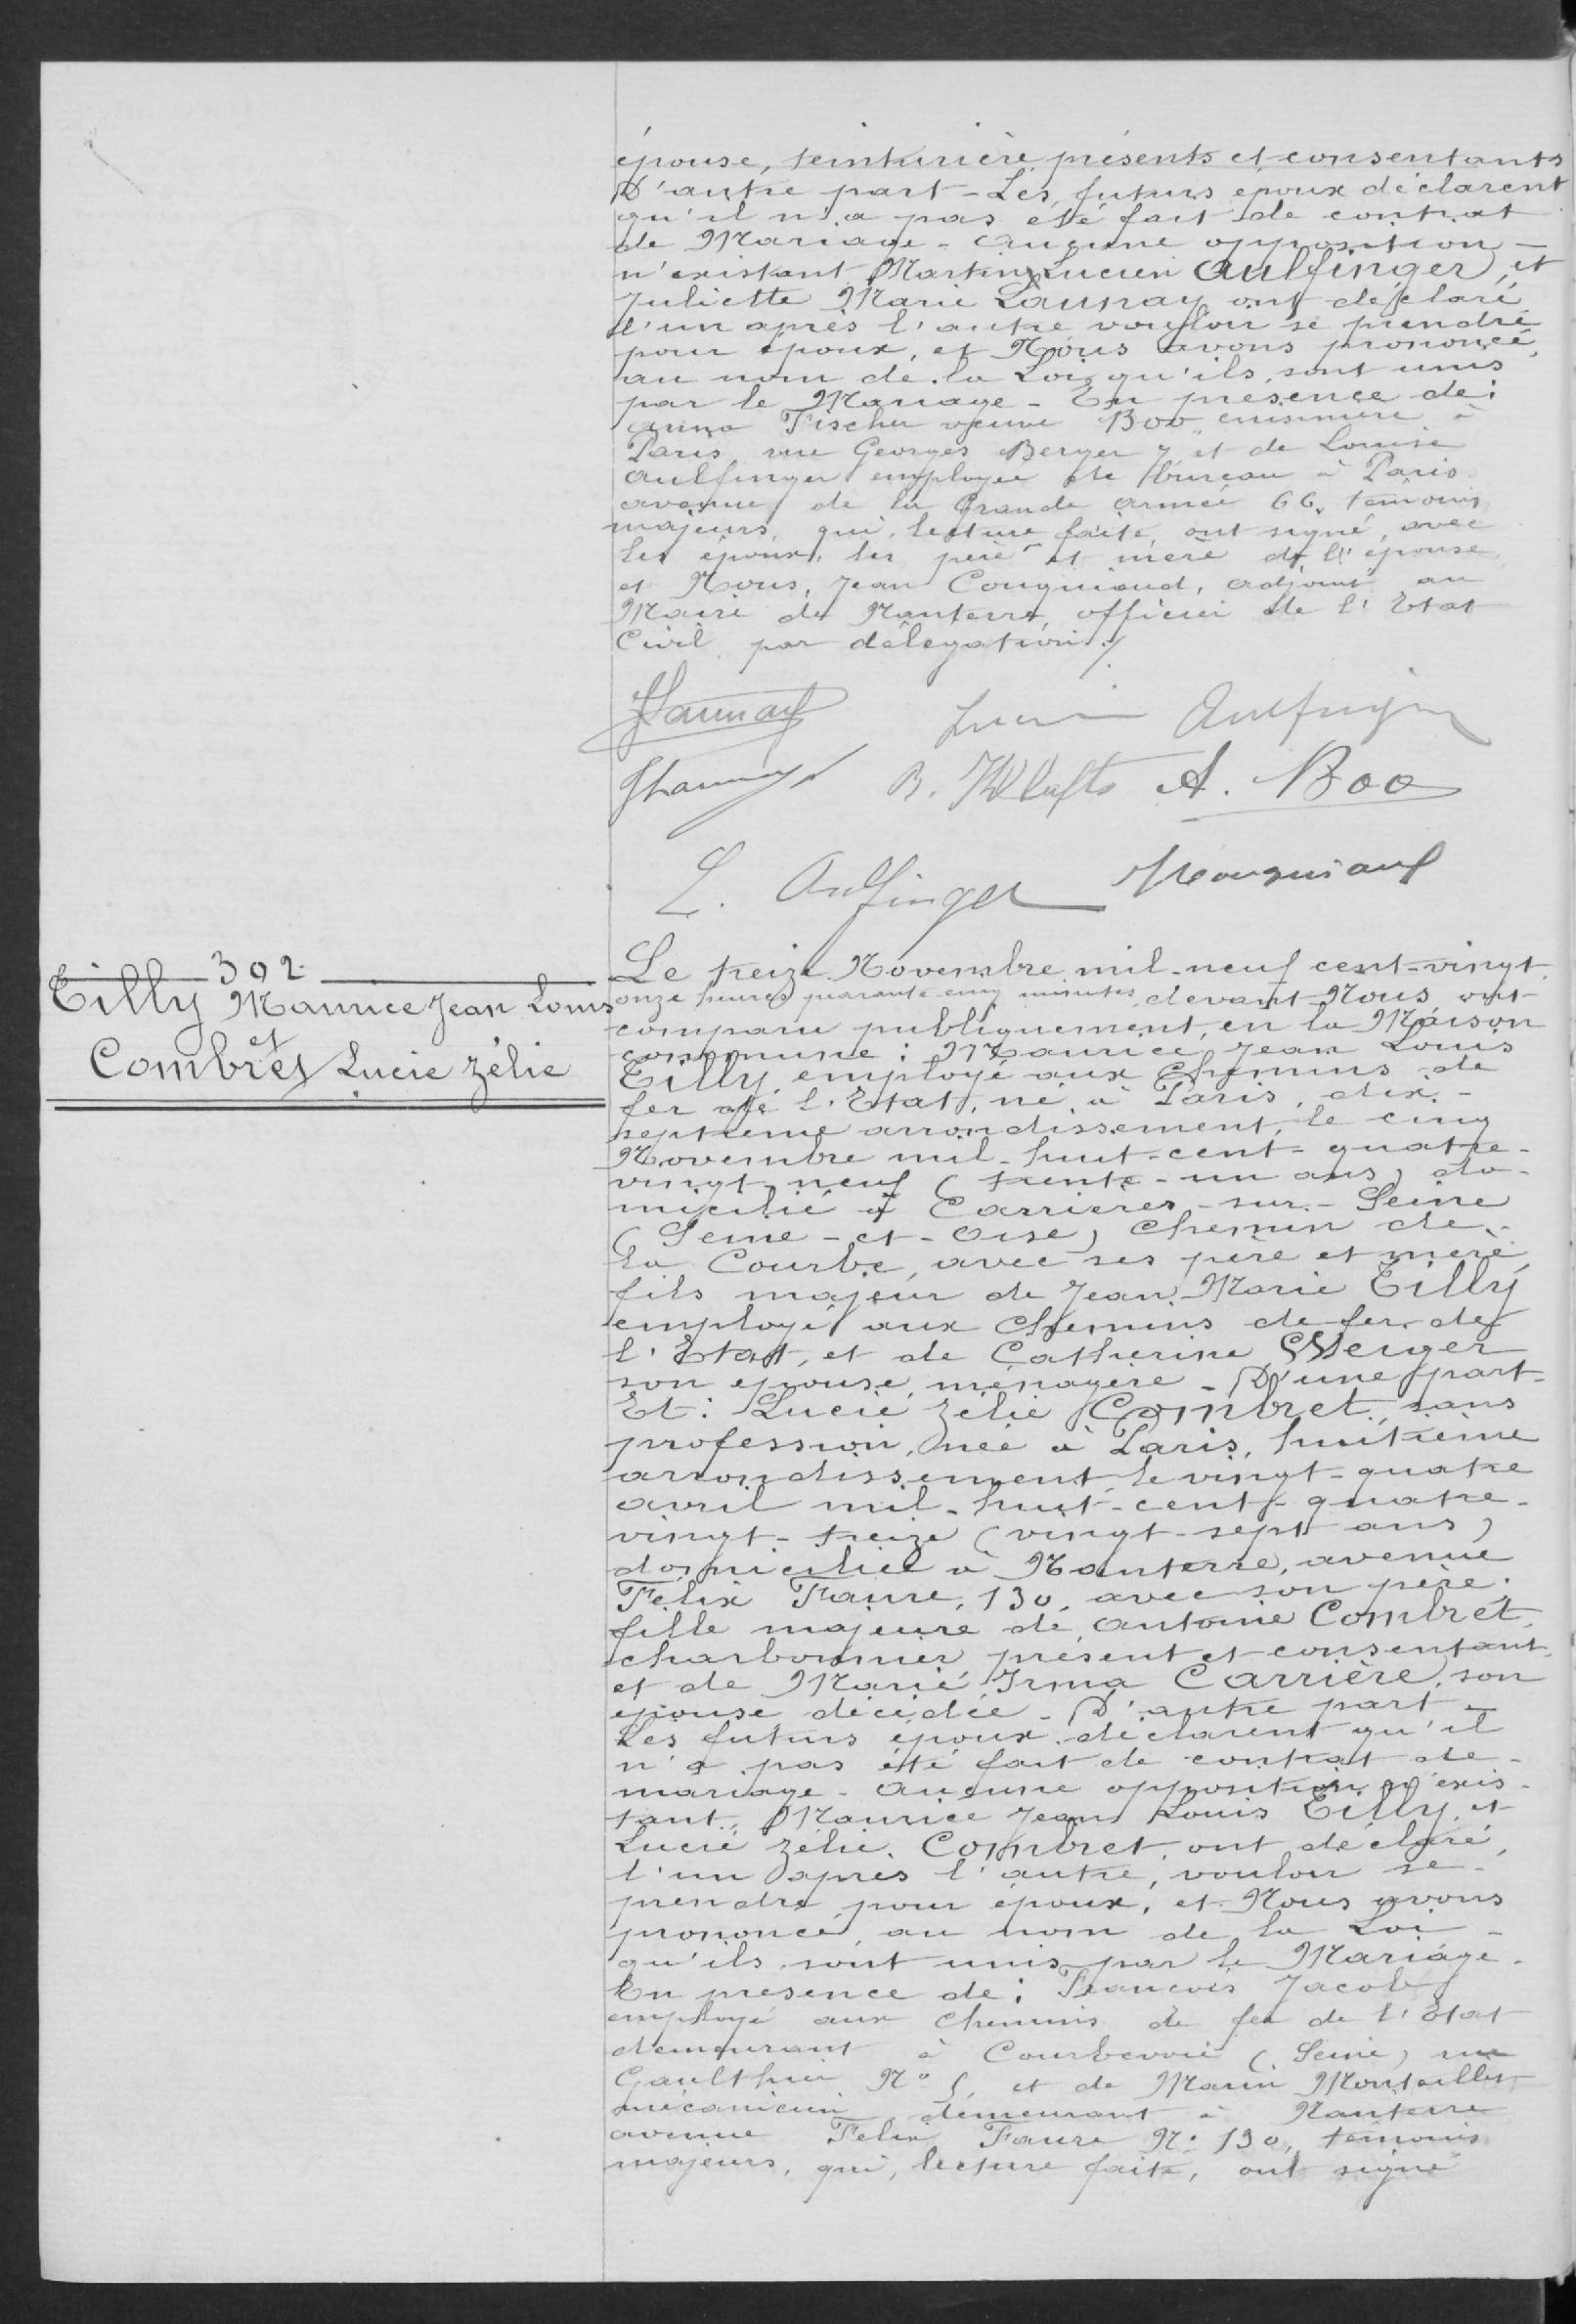épouse, teinturière présents et consentant
D'autre part-Les futurs époux déclarent
qu'il n'a pas été fait de contrat
de Mariage. Aucune opposition-
n'existant Martin Lucien Aulfinger et
Juliette Maire Launay ont déclaré
l'un après l'autre vouloir se prendre
pour époux, et Nous avons prononcé
au nom de la Loi qu'ils sont unis
par le Mariage-En présence de:
arina Fischer veuve Boo cuisinière à
Paris, rue Georges Berger et de Louis
Aulfinger emlpoyé de bureau à Paris
avenue de la Grande armée 66, témoins
majeurs, qui, lecture fait ont signé, avec
les époux, les père et mère de l'épouse
et Nous, Jean Cougniaud, adjoint au
Maire de Nanterre, officier de l'Etat
Civil par délégation./.
302
Cilly Maurice Jean Louis
et
Combres Lucie Zélie
Le treize Novembre mil neuf cent-vingt
onze heure quarante cinq minutes devant Nous ont
comparu publiquement en la Maison
commune: Maurice Jean Louis
Tilly, employé aux chemins de
fer de l'Etat, né à Paris, dix-
septième arrondissement le cinq
Novembre mil huit cent quatre
vingt neuf (trente-un ans) do-
micilié à Carrières-sur-Seine
(Seine-et-Oise) chemin de
la Courbe, avec ses père et mère
fils majeur de Jean Marie Tilly
employé aux chemins de fer de
l'Etat, et de Catherine Meiger
son épouse, ménagère-D'une part
Et Lucie Zélie Coinbret sans
profession, née à Paris huitième
arrondissement le vingt-quatre
avril mil-huit-cent-quatre
vingt-treize (vingt-sept ans)
domicilié à Nanterre avenue
Félix Faure, 130, avec son père
fille majeure de Antoine Combret
charbonnier présent et consentant
et de Marie Irma Carrière son
épouse décédée. D'autre part
Les futurs époux déclarent qu'il
n'a pas été fait de contrat de
mariage. Aucune opposition n'exis
tant Maurice Jean Louis Tilly et
Lucie Zélie Combret ont déclaré
l'un après l'autre vouloir se
prendre pour époux et Nous avons
prononcé au nom de la Loi -
qu'ils sont unis par le Mariage.
En présence de: François Jacob
employé au chemin de fer de l'Etat
demeurant à Courbevoie (Seine) rue Gaulthier N° 5, et de Marie Montaillet
mécanicien demeurant à Nanterre
avenue Félix Faure 92 190 témoins
majeurs, qui, lecture faite, ont signé
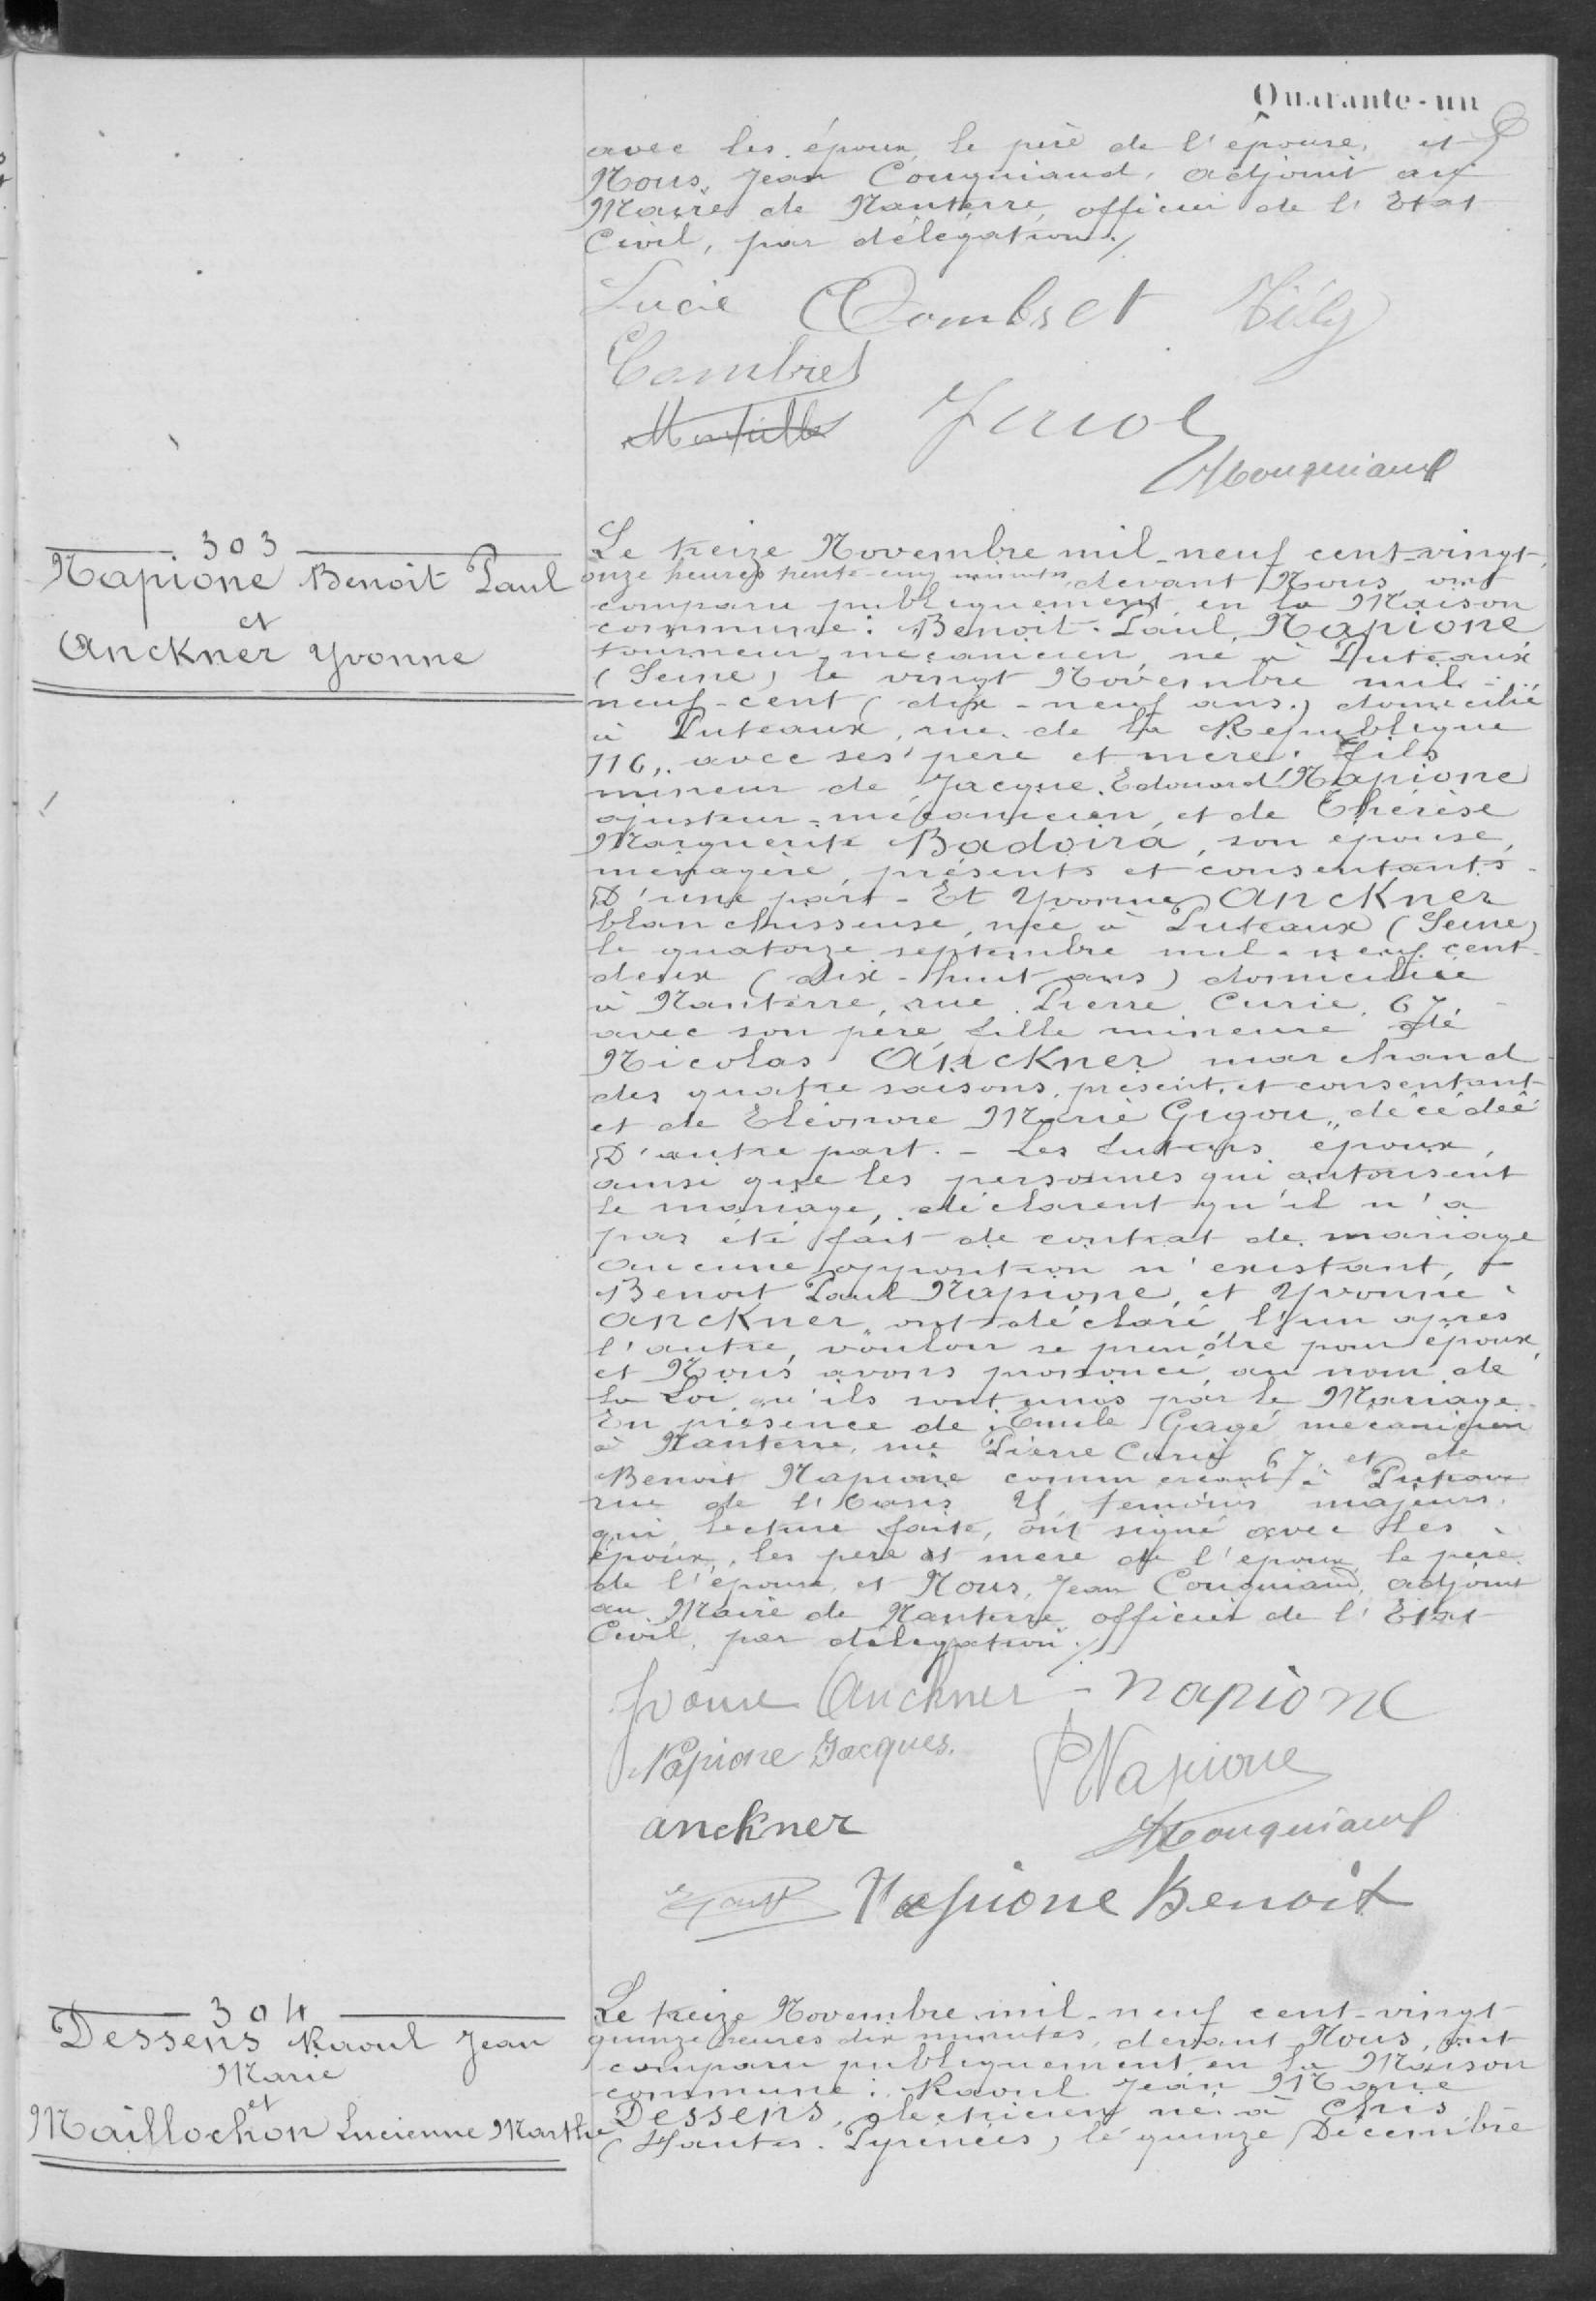avec les époux le père de l'épouse et
nous, Jean Congniard, Adjoint au
Maire de Nanterre, officier de l'Etat
Civil par délégation./.
Le treize Novembre mil neuf cent vingt
onze heure trente cinq minutes, devant Nous ont
comparu publiquement en la Maison
commune: Benoit Paul Mapione
tourneur mecanicien, né à Puteaux
(Seine) le vingt Novembre mil-
neuf-cent (dix-neuf ans) domicilié
à Puteaux, rue de la République
116, avec ses père et mère. Fils
mineur de Jacque Edouard Mapione
ajusteur mécanicien, et de Thérèse
Marguerite Badoira, son épouse,
ménagère, présents et consentants-
D'une part. Et Yvonne Anckner
blanchisseuse, née à Puteaux (Seine)
le quatorze septembre mil neuf cent
deux (dix-huit ans) domiciliée
à Nanterre, rue Pierre Curie 67,
avec son père fille mineure de
Nicolas Anckner marchand
des quatre saisons, présent et consentant
et de Eléonore Marie Grégoire, décédée.
D'autre part.-Les futurs époux,
ainsi que les personnes qui autorisent
le mariage, déclarent qu'il n'a
pas été fait contrat de mariage.
Aucune opposition n'existant,
Benoit Paul Mapione et Yvonne
Anckner, ont déclaré, l'un après
l'autre vouloir se prendre pour époux
et Nous avons prononcé au nom de
La Loi qu'ils sont unis par le Mariage.
En présence de Emile Gagé mécanicien
à Nanterre rue Pierre Cerri 67 et de
Benoit Mapione commercant à Puteaux
qui, lecture faite, ont signé avec les
époux, les père et mère de l'époux le père
de l'épouse et Nous, Jean Congniand adjoint
au Maire de Nanterre, officier de l'Etat
Civil, par délégation.
304
Dessens Raoul Jean
Marie
et
Maillochon Lucienne Marthe
Le treize Novembre mil neuf cent vingt
quinze heures dix minutes devant Nous, ont
comparu publiquement en la Maison
commune: Raoul Jean Magne
Dessens, electricien, né à Chis
(Haute-Pyrénées) le quinze décembre